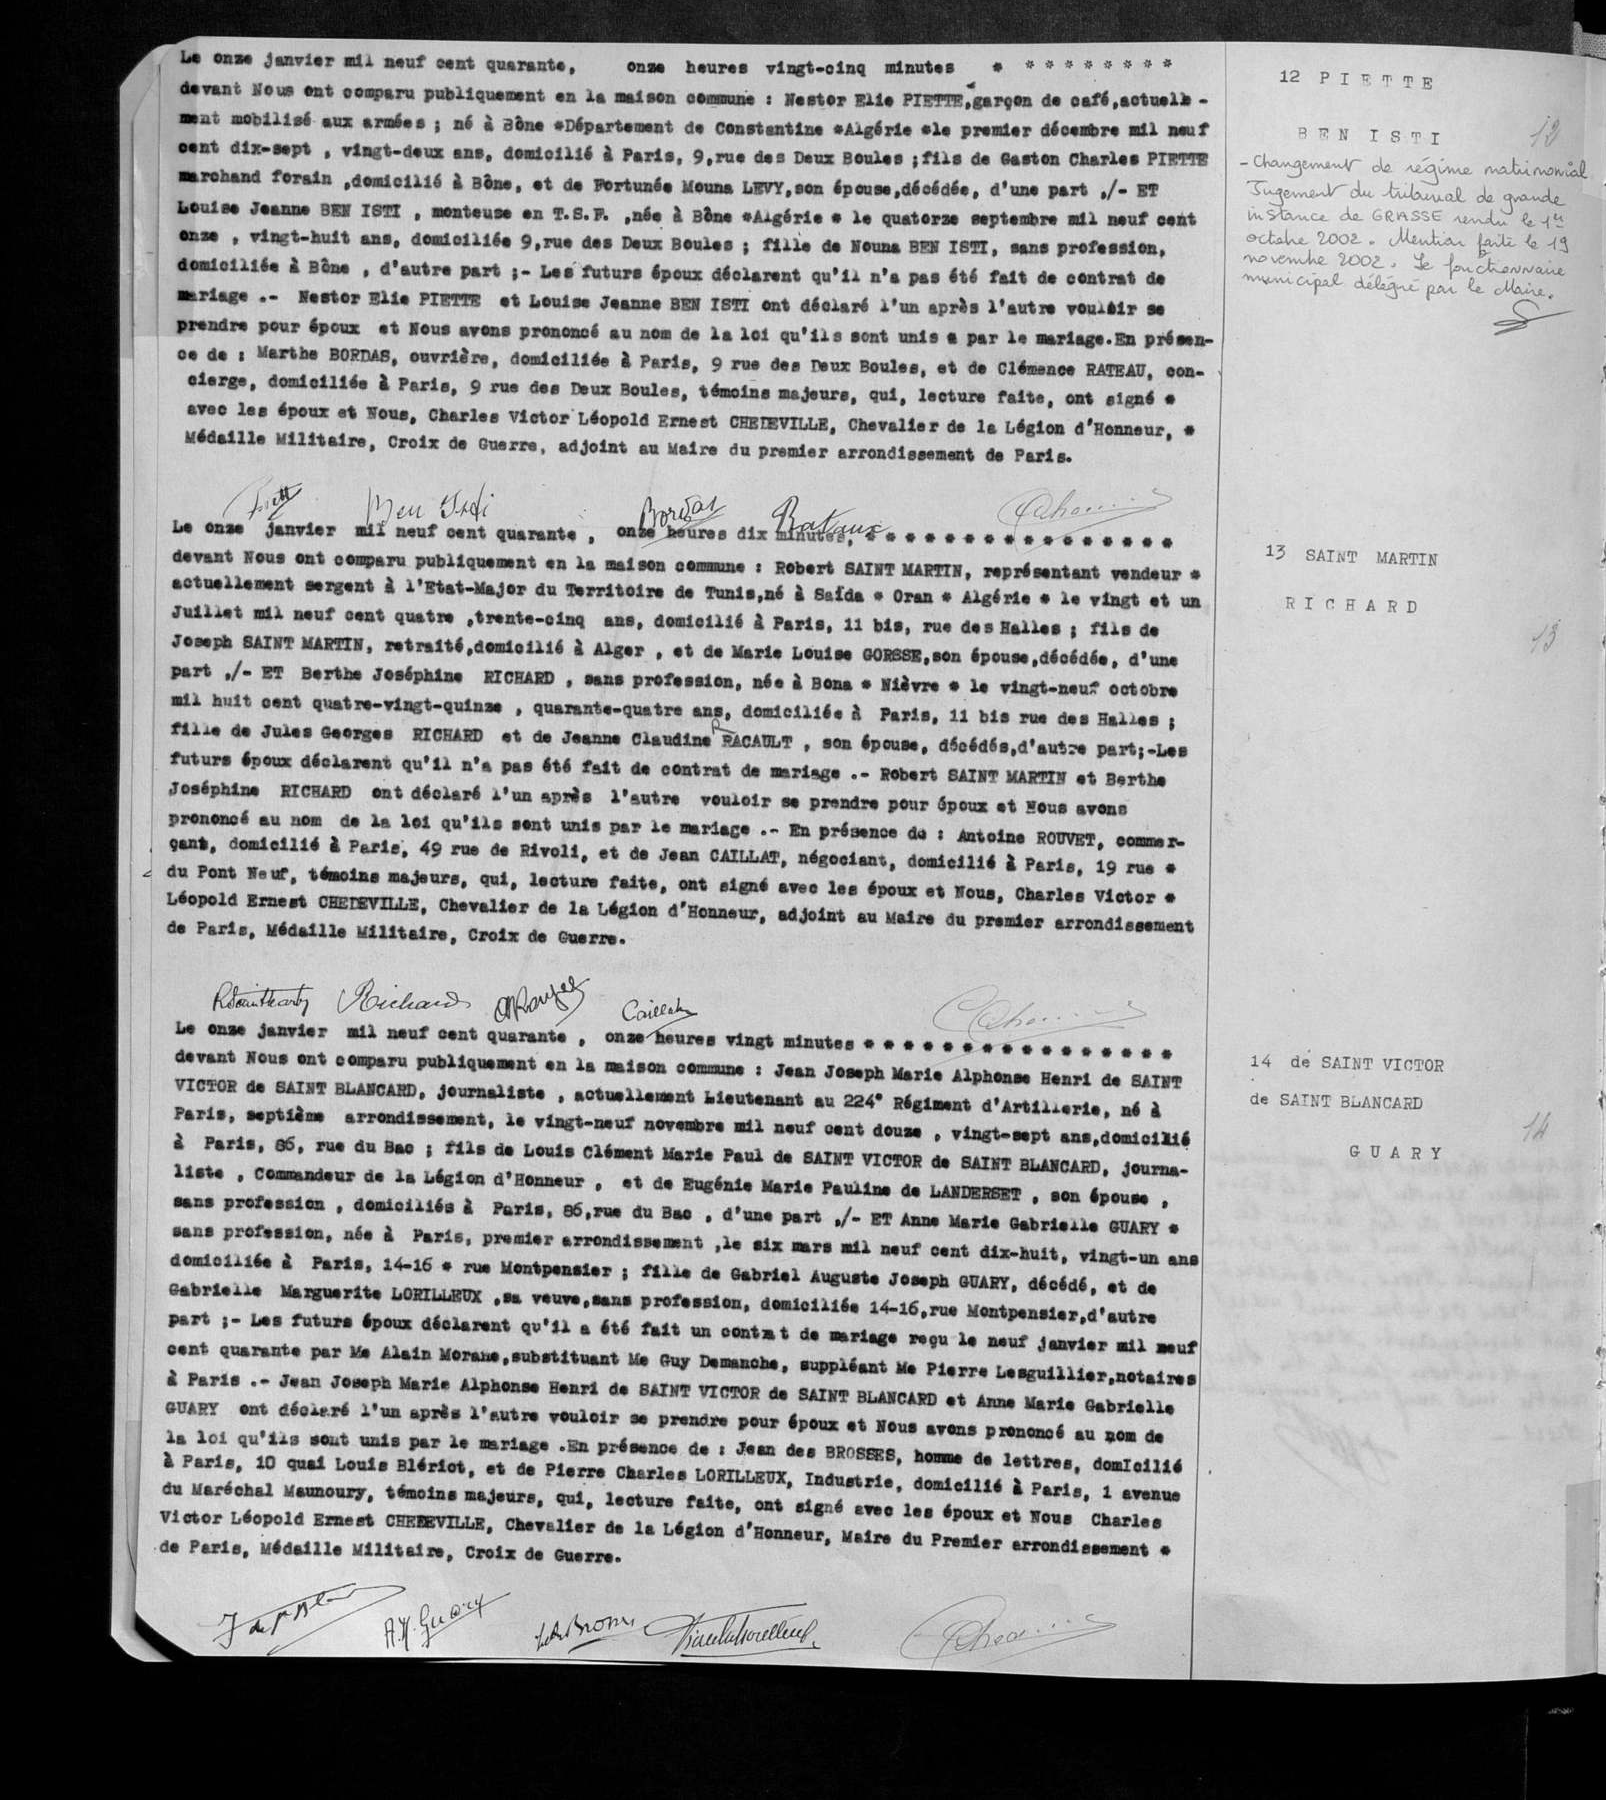Le onze janvier mil neuf cent quarante, onze heures vingt-cinq minutes ****
devant Nous ont comparu publiquement en la maison commune: Nester Elie PIETTE, garçon de café, actuelle-
ment mobilisé aux armées; né à Bône * Département de Constantine * Algérie * le premier décembre mil neuf
cent dix-sept, vingt-deux ans, domicilié à Paris, 9, rue des Deux Boules; fils de Gaston Charles PIETTE
marchand forain, domicilié à Bône, et de Fortunée Moins LEVY, son épouse, décédée, d'une part ,/-ET
Louise Jean BEN ISTI, monteur en T. S. F., née à Bône * Algérie * le quatorze septembre mil neuf cent
onze, vingt-huit ans, domiciliée 9, rue des Deux Boules; fille de Nouna BEN ISTI, sans profession,
domicilié à Bône, d'autre part ;-Les futurs époux déclarent qu'il n'a pas été fait de contrat de
mariage .-Nestor Elie PIETTE et Louise Jeanne BEN ISTI ont déclaré l'un après l'autre vouloir se
prendre pour époux et Nous avons prononcé au nom de la loi qu'ils sont unis * par le mariage. En présen-
ce de: Marthe BORDAS, ouvrière, domiciliée à Paris, 9 rue des Deux Boules, et de Clémence RATEAU, con-
cierge, domiciliée à Paris, 9 rue des Deux Boules, témoins majeurs, qui, lecture faite, ont signé *
avec les époux et Nous, Charles Victor Léopold Ernest CHELEVILLE, Chevalier de la Légion d'Honneur, *
Médaille Militaire, Croix de Guerre, adjoint au Maire du premier arrondissement de Paris.
Le onze janvier mil neuf cent quarante, onze heures dix minutes ****
devant Nous ont comparu publiquement en la maison commune: Robert SAINT MARTIN, représentant vendeur *
actuellement sergent à l'Etat-Major du Territoire de Tunis, né à Saïda * Oran * Algérie * le vingt et un
Juillet mil neuf cent quatre, trente-cinq ans, domicilié à Paris, 11 bis, rue des Halles; fils de
Joseph SAINT MARTIN, retraité, domicilié à Alger, et de Marie Louise GORSSE, son épouse, décédée, d'une
part, ET Berthe Joséphine RICHARD, sans profession, née à Bons * Nièvre * le vingt-neuf octobre
mil huit cent quatre-vingt-quinze, quarante-quatre ans, domiciliée à Paris, 11 bis rue des Halles;
fille de Jules Georges RICHARD et de Jeanne Claudine RACAULT, son épouse, décédée, d'autre part ;-Les
futurs époux déclarent qu'il n'a pas été fait de contrat de mariage .-Robert SAINT MARTIN et Berthe
Joséphine RICHARD ont déclaré l'un après l'autre vouloir se prendre pour époux et Nous avons
prononcé au nom de la loi qu'ils sont unis par le mariage .-En présence de: Antoine ROUVET, commer-
cant, domicilié à Paris, 49 rue de Rivoli, et de Jean CAILLAT, négociant, domicilié à Paris, 19 rue *
du Pont Neuf, témoins majeurs, qui, lecture faite, ont signé avec les époux et Nous, Charles Victor *
Léopold Ernest CHELEVILLE, Chevalier de la Légion d'Honneur, adjoint au Maire du premier arrondissement
de Paris, Médaille Militaire, Croix de Guerre.
Le onze janvier mil neuf cent quarante, onze heures vingt minutes ****
devant Nous ont comparu publiquement en la maison commune: jean Joseph Marie Alphonse Henri de SAINT
VICTOR de SAINT BLANCARD, journaliste, actuellement Lieutenant au 224° Régiment d'Artillerie, né à
Paris, septième arrondissement, le vingt-neuf novembre mil neuf cent douze, vingt-sept ans, domicilié
à Paris, 06, rue du Bac; fils de Louis Clément Marie Paul de SAINT VICTOR de SAINT BLANCARD, journa-
liste, Commandeur de la Légion d'Honneur, et de Eugénie Marie Pauline de LANDERSET, son épouse,
sans profession, domiciliés à Paris, 86, rue du Bac, d'une part ,/-ET Anne Marie Gabrielle GUARY *
sans profession, née à Paris, premier arrondissement, le six mars mil neuf cent dix-huit, vingt-un ans
domiciliée à Paris, 14-16 * rue Montpellier; fille de Gabriel Auguste Joseph GUARY, décédé, et de
Gabrielle Marguerite LORILLEUX, sa veuve, sans profession, domiciliée 14-16, rue Montpensier, d'autre
part ;-Les futurs époux déclarent qu'il a été fait un contat de mariage reçu le neuf janvier mil neuf
cent quarante par Me Alain Morane, substituant Me Guy Dimanche, suppléant Me Pierre Lesguillier, notaires
à Paris .-Jean Joseph Marie Alphonse Henri de SAINT VICTOR de SAINT BLANCARD et Anne Marie Gabrielle
GUARY ont déclaré l'un après l'autre vouloir se prendre pour époux et Nous avons prononcé au nom de
la loi qu'ils sont unis par le mariage. En présence de: Jean des BROSSES, homme de lettres, domIcilié
à Paris, 10 quai Louis Blériot, et de Pierre Charles LORILLEUX, Industrie, domicilié à Paris, 1 avenue
du Maréchal Maunoury, témoins majeurs, qui, lecture faite, ont signé avec les époux et Nous Charles
Victor Léopold Ernes CHELEVILLE, Chevalier de la Légion d'Honneur, Maire du Premier arrondissement *
de Paris Médaille Militaire, Croix de Guerre.
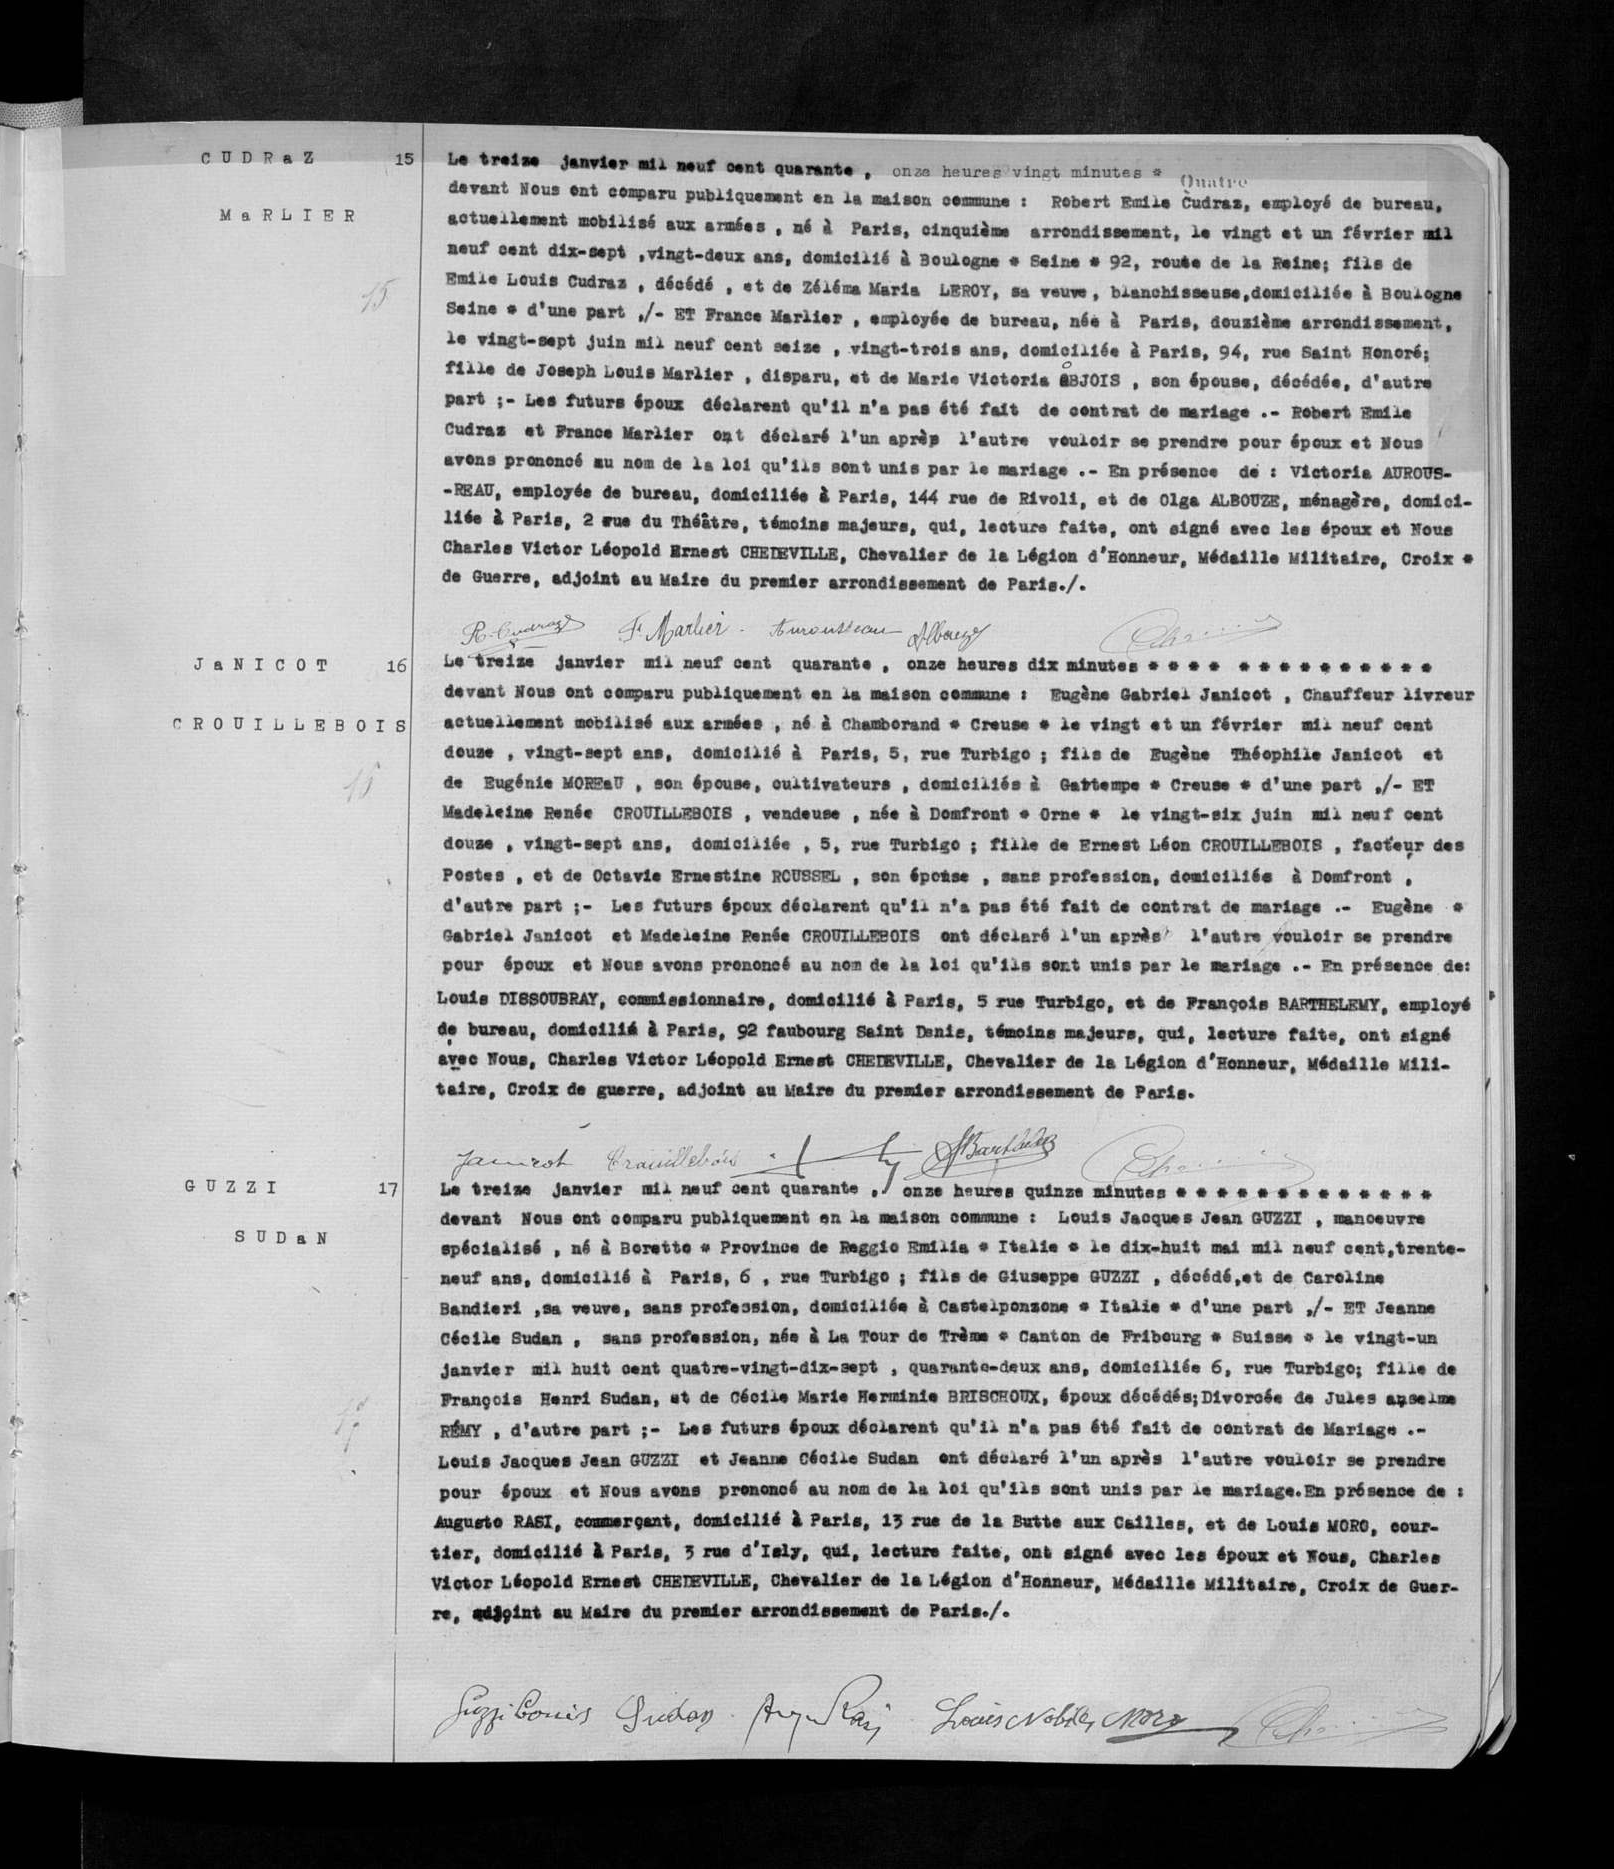Le treize janvier mil neuf cent quarante, onze heures vingts minutes *
devant Nous ont comparu publiquement en la maison commune: Robert Emile Cudras, employé de bureau,
actuellement mobilisé aux armées, né à Paris, cinquième arrondissement, le vingt et un février mil
neuf cent dix-sept, vingt-deux ans, domicilié à Boulogne * Seine * 92, rouée de la Reine; fils de
Emilé Louis Cudras, décédé, et de Zéléma Maria LEROY, sa veuve, blanchisseuse domiciliée à Boulogne
Seine * d'une part ,/-ET France Marlier, employée de bureau, née à Paris, douzième arrondissement,
le vingt-sept juin mil neuf cent seize, vingt-trois ans, domiciliée à Paris, 94, rue Saint Honoré;
fille de Joseph Louis Marlier, disparu, et de Marie Victoria OBJOIS, son épouse, décédée, d'autre
part ;-Les futurs époux déclarent qu'il n'a pas été fait de contrat de mariage .-Robert emile
Cudras et France Marlier ont déclaré l'un après l'autre vouloir se prendre pour époux et Nous
avons prononcé au nom de la loi qu'ils sont unis par le mariage .-En présence de: Victoria AUROUS-
REAU, employée de bureau, domiciliée à Paris, 144 rue de Rivoli, et de Olga ALBOUZE, ménagère, domici-
liée à Paris, 2 rue du Théâtre, témoins majeurs, qui, lecture faite, ont signé avec les époux et Nous
Charles Victor Léopold Ernest CHELEVILLE, Chevalier de la Légion d'Honneur, Médaille Militaire, Croix *
de Guerre, adjoint au Maire du premier arrondissement de Paris ./.
Le treize janvier mil neuf cent quarante, onze heures dix minutes ****
devant Nous ont comparu publiquement en la maison commune: Eugène Gabriel Janicet, Chauffeur livreur
actuellement mobilisé aux armées, né à Chamborand * Creuse * le vingt et un février mil neuf cent
douze, vingt-sept ans, domicilié à Paris, 5, rue Turbigo; fils de Eugène Théophile Janicot et
de Eugénie MOREaU, son épouse, cultivateurs, domiciliés à Gattempe * Creuse * d'une part ,/-ET
Madeleine Renée CROUILLEBOIS, vendeuse, née à Domfront * Orne * le vingt-six juin mil neuf cent
douze, vingt-sept ans, domiciliée, 5, rue Turbigo; fille de Ernest Léon CROUILLEBOIS, facteur des
Postes, et de Octavie Ernestine ROUSSEL, son épouse, sans profession, domiciliés à Domfront,
d'autre part ;-Les futurs époux déclarent qu'il n'a pas été fait de contrat de mariage .-Eugène *
Gabriel Janicet et Madeleine Renée CROUILLEBOIS ont déclaré l'un après l'autre vouloir se prendre
pour époux et Nous avons prononcé au nom de la loi qu'ils sont unis par le mariage .-En présence de:
Louis DISSOUBRAY, commissionnaire, domicilié à Pairs, 5 rue Turbigo, et de François BARTHELEMY, employé
de bureau, domicilié à Paris, 92 faubourg Saint Denis, témoins majeurs, qui, lecture faite, ont signé
avec Nous, Charles Victor Léopold Ernest CHELEVILLE, Chevalier de la Légion d'Honneur, Médaille Mili-
taire, Croix de guerre, adjoint au Maire du premier arrondissement de Paris.
Le treize janvier mil neuf cent quarante, onze heures quinze minutes ****
devant Nous ont comparu publiquement en la maison commune: Louis Jacques Jean GUZZI, manoeuvre
spécialisé, né à Borette * Province de Reggie Emilie * Italie * le dix-huit mai mil neuf cent, trente-
neuf ans, domicilié à Paris 6, rue Turbigo; fils de Giusseppe GUZZI, décédé, et de Caroline
Bandierie, sa veuve, sans profession, domiciliée à Castelponzone * Italie * d'une part ,/-ET Jeanne
Cécile Sudan, sans profession, née à la Tour de Trème * Canton de Fribourg * Suisse * le vingt-un
janvier mil huit cent quatre-vingt-dix-sept, quarante-deux ans, domiciliée 6, rue Turbigo; fille de
François Henri Sudan, et de Cécile Marie Herminie BRISCHOUX, époux décédés; Divorcée de Jules anselme
REMY, d'autre part ;-Les futurs époux déclarent qu'il n'a pas été fait de contrat de Mariage .-
Louis Jacques Jean GUZZY et Jeanne Cécile Sudan ont déclaré l'un après l'autre vouloir se prendre
pour époux et Nous avons prononcé au nom de la loi qu'ils sont unis par le mariage. En présence de:
Auguste RASI, commerçant, domicilié à Paris, 13 rue de la Butte aux Cailles, et de Louis MORO, cour-
tier, domicilié à Paris, 3 rue d'Isly, qui, lecture faite, ont signé avec les époux et Nous, Charles
Victor Léopold Ernest CHELEVILLE, Chevalier de la Légion d'Honneur, Médaille Militaire, Croix de Guer-
re, adjoint au Maire du premier arrondissement de Paris ./.